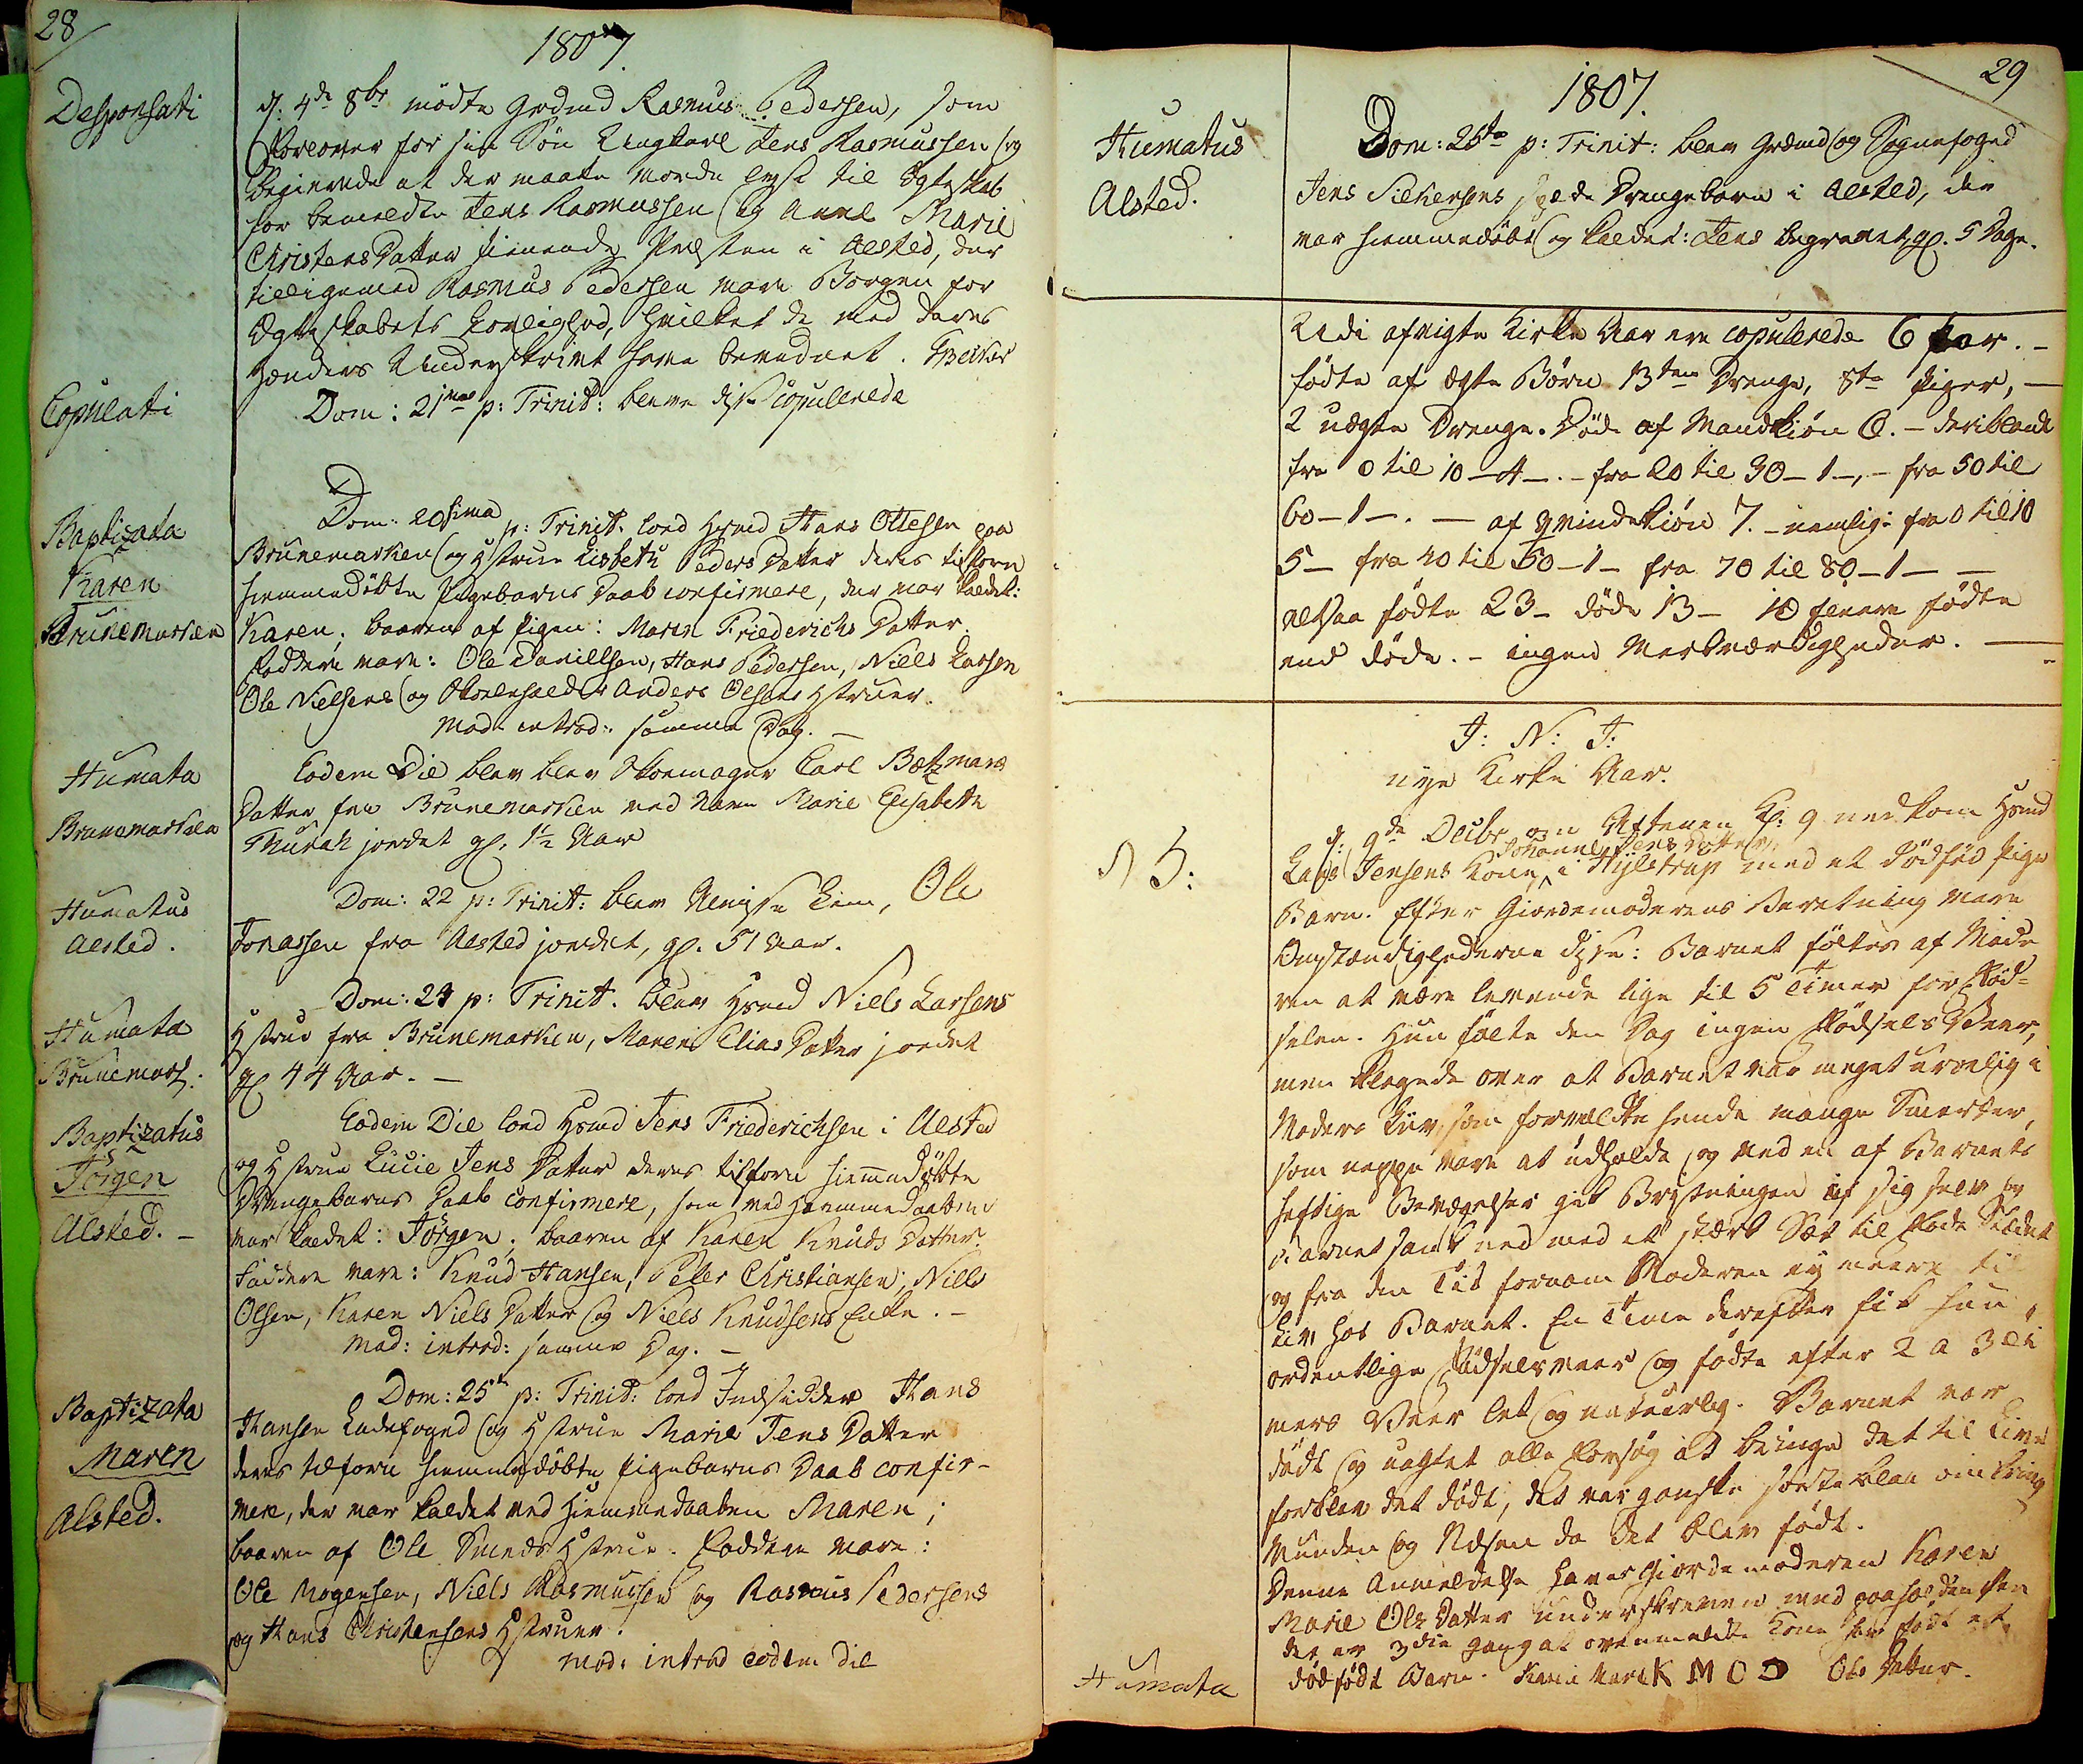28.
Deponsati
Copulati
Baptizata
Karen
Brunemarken
Humata
Brunemarken
Humatus
Alsted
Humata
Brunemark:
Baptizatus
Jørgen
Alsted.
Baptizata
Maren
Alsted.
1807.
d: 4de 8br mødte Grdmd Rasmus Pedersen, som
Forlover for sin Søn Ungkarl Jens Rasmussen og
begierede at der maate vorde lyst til Ægteskab
for bemeldte Jens Rasmussen og Anne Marie
Christens datter tienende Præsten i Alsted, der
tilligemed Rasmus Pedersen vare boren for
Ægteskabets Lovlighed, hvilket de med deres
Hænders Underskrivt have bevidnet. G Becker
Dom: 21ma p: Trinit: bleve disse copulerede
Dom: 20sima p: Trinit: loed Hsmd Hans Ottesen paa
Brunemarken og Hstrue Lisbeth Peders Datter deris tilforn
hiemmedøbte Pigebarns Daab Confirmere, der var kaldet:
Karen; baaren af Pigen: Maren Friderichs Datter
Faddere vare: Ole Danielsen, Hans Pedersen, Niels Larsen
Ole Nielsens og Skoleholder Anders Olsens Hstruer
Mod: introd: samme Dag.
Eodem Die blev blev Skoemager Carl Bætzmans
Datter fra Brunemarken ved Navn Marie Elisabeth
Thurah jordet gl: 1½ Aar
Dom: 22 p: Trinit: blev Almisse Lem, Ole
Jonassen fra Alsted jordet, gl: 51 Aar.
Dom: 24 p: Trinit. blev Hsmd Niels Larsens
Hstrue fra Brunemarken, Maren Elias Datter jordet
gl 44 Aar.
Eodem Die loed Hsmd Jens Friderichsen i Alsted
og Hstrue Lucie Jens Datter deres tilforn hiemmedøbte
Drengebarns Daab confirmere, som ved Hiemmedaaben
var kaldet: Jørgen. baaren af Karen Knuds Datter
Faddere vare: Knud Hansen, Peter Christiansen, Niels
Olsen, Karen Niels Datter og Niels Knudsens Enke.
Mod: introd: samme Dag.
Dom: 25de p: Trinit: loed Indsidder Hans
Hansen Ladefoged og Hstrue Marie Jens Datter
deres tilforn hiemmedøbte Pigebarns Daab confir¬
mere, der var kaldet ved Hiemmedaaben Maren;
baaren af Ole Smeds Hstrue. Faddere vare:
Ole Mogensen, Niels Rasmussen og Rasmus Pedersens
og Hans Christensens Hustruer.
Mod: introd Eodem Die
Humatus
Alsted.
NB:
Humata
29.
1807.
Dom: 25ta p: Trinit: blev Grdmd og Sognefoged
Jens Silkensens spæde Drengebarn i Alsted, der
var hiemmedøbt og kaldet: Jens begravet gl. 5 Dage.
Udi afvigte Kirke Aar ere copulerede 6 Par.
fødte af ægte Børn 13ta## Drenge, 8ta Piger,
2 uægte Drenge. Døde af Mandkiøn 6. - deriblandt
fra 0 til 10 - 4 - . - fra 20 til 30 - 1 -, - fra 50 til
60 - 1 - af Qvindekiøn 7. nemlig: fra 0 til 10
5 - fra 40 til 50 - 1 - fra 70 til 80 - 1 -
altsaa fødte 23 - døde 13 - 10 flere fødte
end døde. - ingen Merkværdigheder.
J: N: J:
nye Kirke Aar.
d: 9de Decbr om Aftenen Kl: 9 nedkom Hsmd
Johanne Jens Datter
Lars Jensens Kone i Hylstrup med et dødfød Pige
Barn. Efter Giordemoderens Beretning vare
Omstændighederen disse: Barnet føltes af Mode
ren at være levende lige til 5 Timer for Fød¬
selen. Hun følte den Dag ingen Fødsels Veer
men klaghede over at Barnet var meget uroelig i
Moders Liv, som forvoldte hende mange Smerter,
som neppe vare at udholde og ved en af Barnets
heftige Bevægelse gik Bristningen af sig selv og
Barnet sank ned med et stært Sæt til Føde Stædet
og fra den Tid formam Moderen ey meere til
Liv hos Barnet. En Time derefter fik hun
ordentligge Fødselsveer og fødte efter 2 a 3 Ti
mers Veer let og naturlig. Barnet var
dødt og uagtet alle Forsøg at bringe det til Live
forblev det dødt, det var ganske sorte blaa omkring
Munden og Næsen da det blev født.
Denne Anmeldse haver Giordemoderen Karen
Marie Olsdatter underskreven med paaholden Pen
det er 3die Gang at ovenmelde Kone har født af
dødfødt Barn. Karen Marie K M O D Ols Datter.
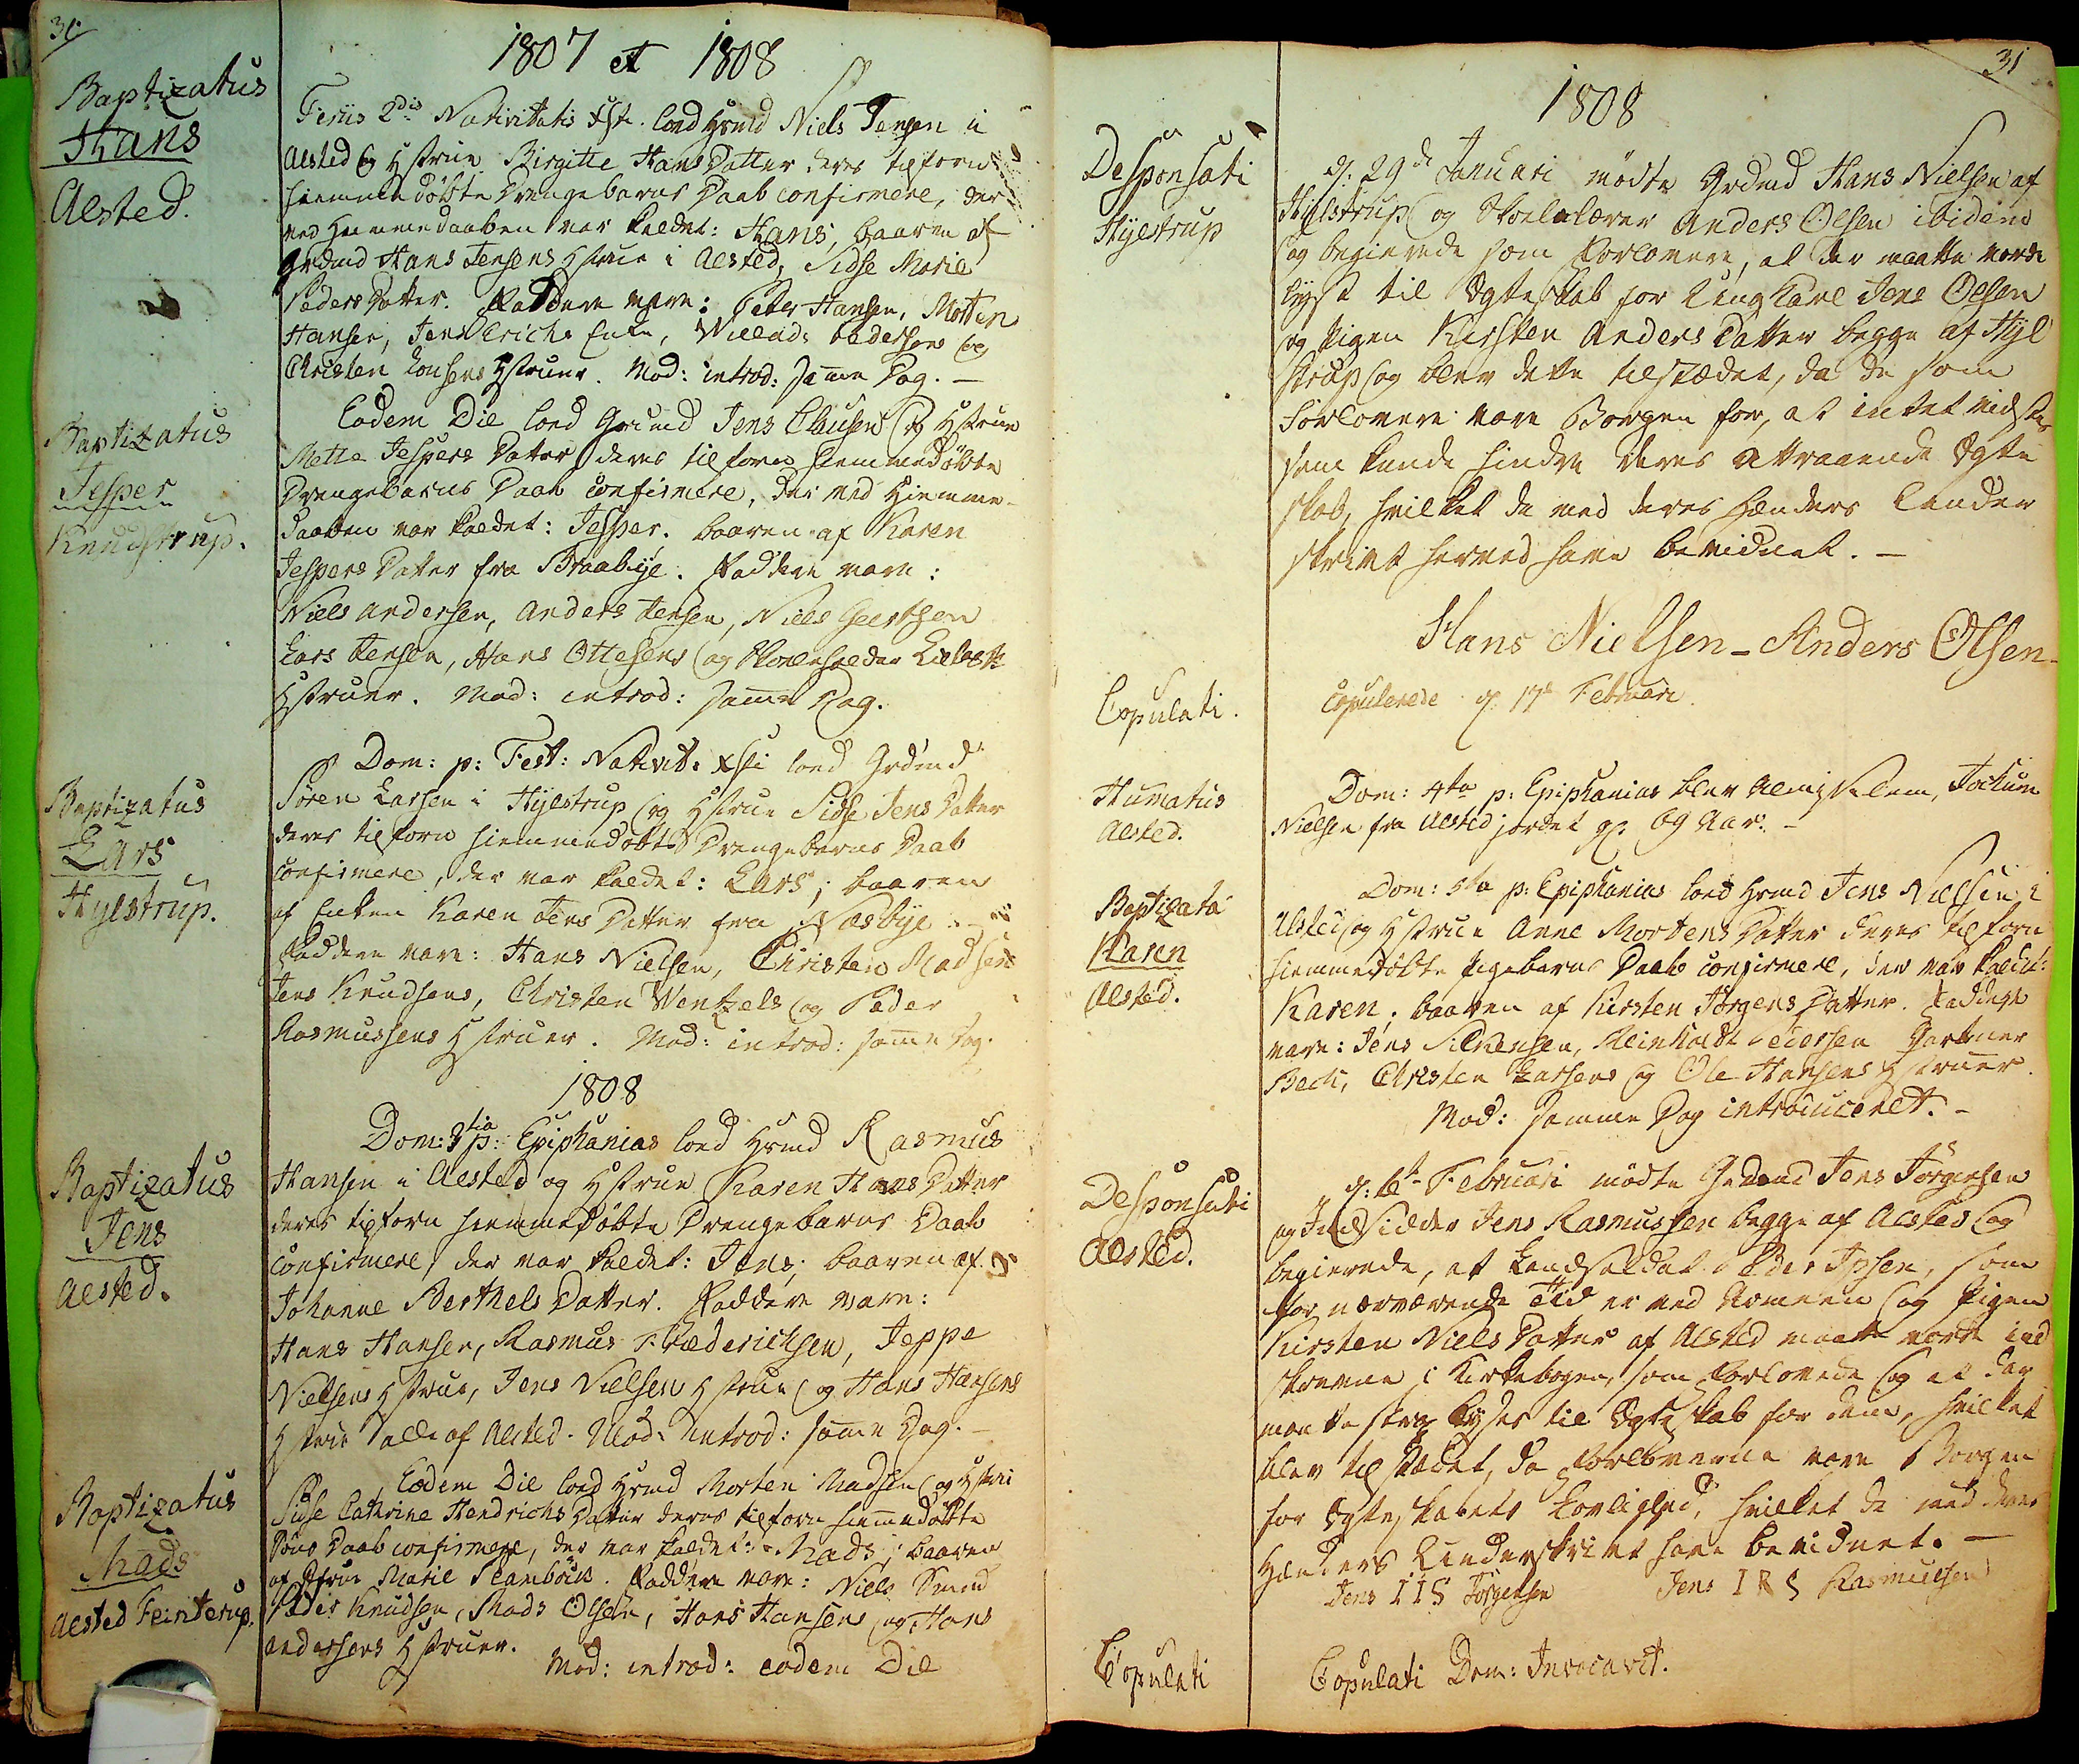30
Baptizatus
Hans
Alsted.
Baptizatus
Jesper
Knudstrup.
Baptizatus
Lars
Hylstrup.
Baptizatus
Jens
Alsted.
Baptizatus
Mads
Alsted Flinterup.
1807 et 1808
Feriis 2dis Nativitatis Xsti. loed Hsmd Niels Jensen i
Alsted og Hstrue Birgitte Hans Datter deres tilforn
hiemmedøbte Drengebarns Daab confirmere, der
ved Hiemmedaaben var kaldet: Hans; baaren af
Grdmd Hans Jensens Hstrue i Alted, Sidse Marie
Peders Datter. Faddere vare: Peder Hansen, Morten
Hansen, Jens Erichs Enke, Willads Pedersen og
Christen Lonsens Hstruer. Mod: introd: samme Dag.
Eodem Die loed Grdmd Jens Clausen og Hstrue
Mette Jespers Datter deres tilforn hiemmedøbte
Drengebarns Daab confirmere, der med Hiemme¬
daaben var kaldet: Jesper. baaren af Karen
Jespers Datter fra Braabye. Faddere vare:
Niels Anderssen, Anders Jensen, Niels Geertsen
Lars Jensen, Hans Ottesens og Skoeleholder Liebsts
Hstruer. Mod: introd: samme Dag.
Dom: p: Fest: Nativit: Xsti loed Grdmd
Søren Larsen i Hylstrup og Hstrue Sidse Jens Datter
deres tilforn hiemmedøbt Drengebarns Daab
confirmere, der var kaldet: Lars;baaren
af Enken Karen Jens Datter fra Næsbye
Faddere vare: Hans Nielsen, Christen Madsen
Jens Knudsens, Christen Wentzels og Peder
Rasmussens Hstruer. Mod: introd: samme Dag.
1808
tia
Dom: 9 p: Epiphanias loed Hsmd Rasmus
Hansen i Alsted og Hstrue Karen Hans Datter
deres tilforn hiemmedøbte Drengebarns Daab
confirmere, der var kaldet: Jens. baaren af
Johanne Berthels Datter. Faddere vare:
Hans Hansen, Rasmus Fræderichsen, Jeppe
Nielsens Hstrue, Jens Nielsen Hstrue og Hans Hansens
Hstrue alle af Alsted. Mod: introd: samme Dag.
Eodem Die loed Hsmd Morten Madsens og Hstri
Sidse Cathrine Hendrichs Datter deres tilforn hiemmedøbte
Søns Daab confirmere, der var kaldet Mads, baaren
af Jfrue Marie Slambøus. Faddere vare: Niels Smed
Peder Knudsen, Mads Olsen, Hans Hansens og Hans
Andersøns Hstruer.
Mod: introd: eodem Die
Desponsati
Nyestrup
Copulati
Humatus
Alsted.
Baptizata
Karen
Alsted.
Desponsati
Tested.
Copulati
31
1808
d. 29de Januari mødte Grdmd Hans Nielsen af
Hylstrup og Skoelelærer Anders Olsen ibidem
og begierede som Forlovere, at der maatte vorde
lyst til Ægteskab for Ungkarl Jens Olsen
og Pigen Kirsten AndersDatter begge af Hyl
strup og blev dette tilstædet, da de som
Forlovere vare Borgen for, at intet vidstes
som kunde hindre deres attraaende Ægte
skab, hvilket de med deres Hænders Under
skrivt herved have bevidnet.
Hans Nielsen - Anders Olsen
copulerede d: 17 Februari
Dom: 4ta p: Epiphanias blev Almisselen, Jochum
Nielsen fra Alsted jordet gl: 69 Aar.
Dom: 5ta p: Epiphanias loed Hsmd Jens Nielsen i
Alslef og Hstrue Anne Mortens Datter deres tilforn
hiemmedøbte Pigebarns Daab confirmere, der var kaldt:
Karen; baaren af Kirsten Jørgens Datter. Faddere
vare: Jens Silkensen, Reinholdt Pedersen Gartner
Bech, Christen Larsens og Ole Hansens Hstruer.
Mod. Samme Dag introduceret.
d: 6te Februari mødte Grdmd Jens Jørgensen
og Jndsidder Jens Rasmussen begge af Alsted og
begierede, af Landsoldat Peder Ipsen, som
for nærværende Tid er ved Armeen og Pigen
Kirsten Niels Datter af Alsted maatte vorde ind
skrevne i Kirkeborgen, som forlovere og at der
maate strax lyses til Ægteskab for dem, hvilket
blev tilstædet, da Forloverne vare Borgen
for Ægteskabets Lovlighed, hvilket de med deres
Hænders Underskrivt have bevidnet.
Jens I I S Jørgensen Jens I R S Rasmusen
Copulati Dom: Invocavit.